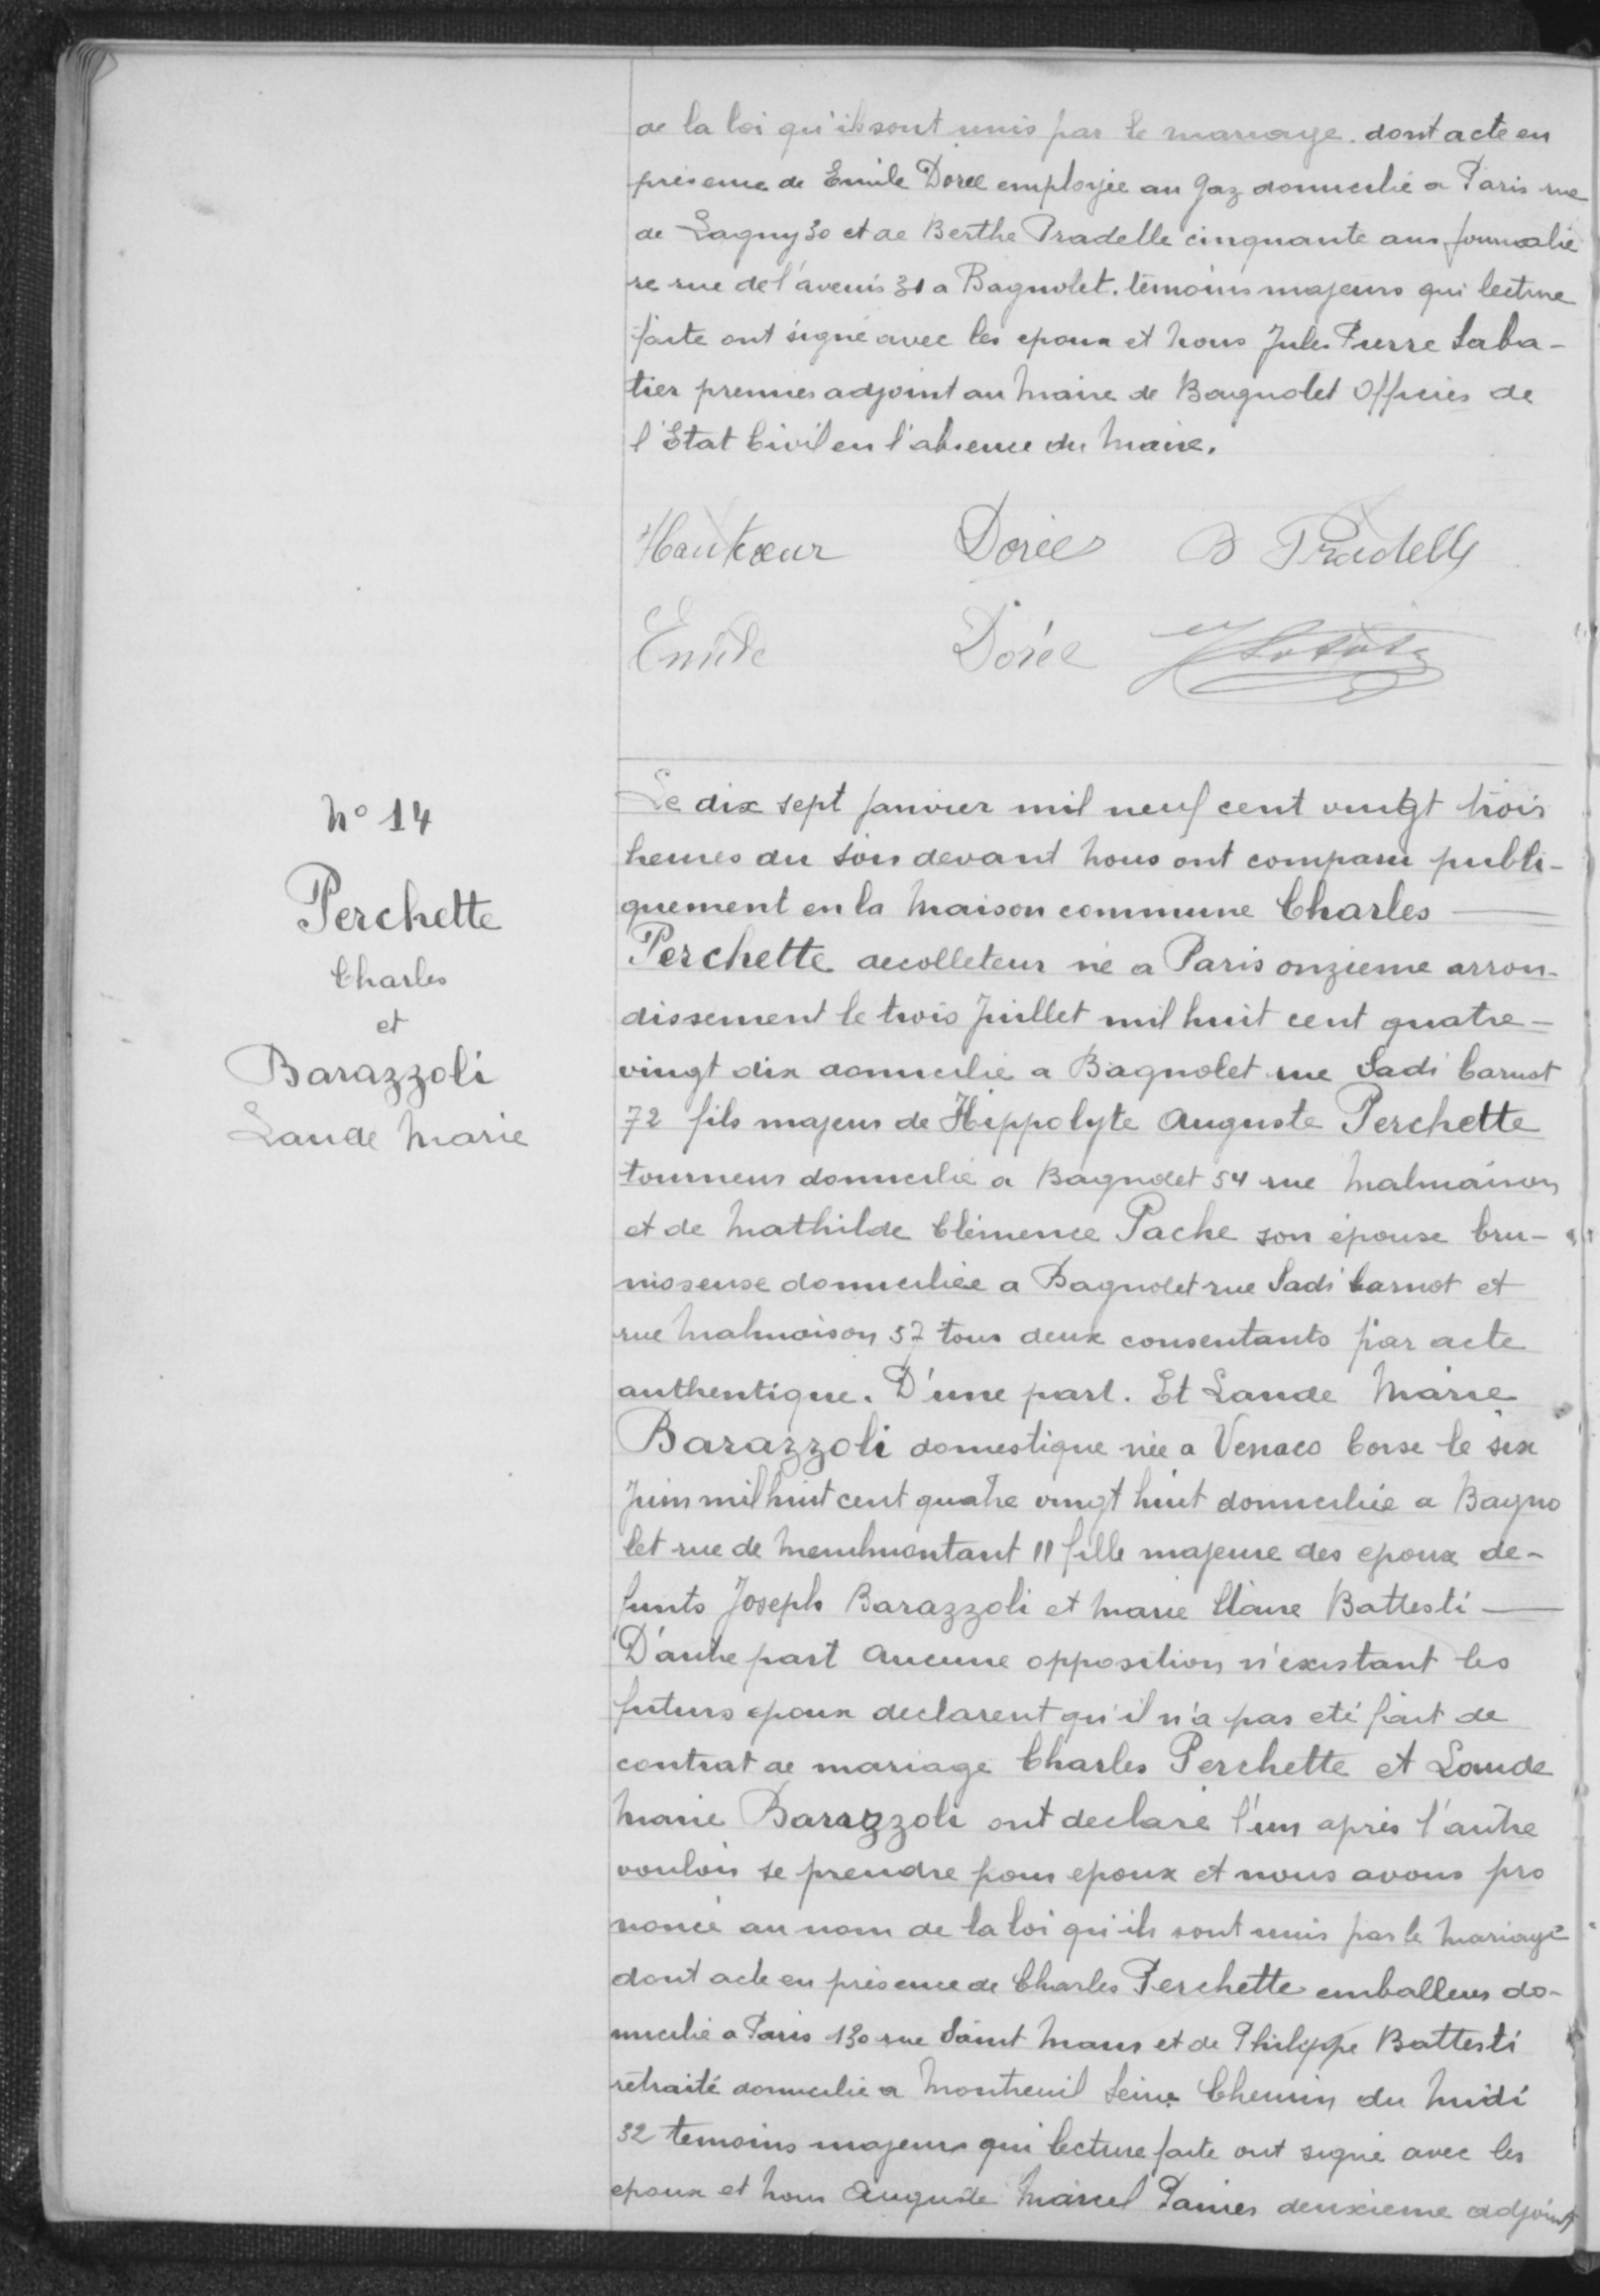a la loi qu'ils sont unis par le mariage, dont acte e
présence de Emile Doree emplloyé au gaz domicilié à Paris rue
de Laguy 30 et ae Berthe Pradelle cinquante ans journaliè
re rue de l'avenis 31 a Bagnolet, temoins majeurs qui lecture
faite ont signé avec les epoux et Nous Jules Pierre Saba-
tier premier adjoint au Maire de Bagnolet Officier de
l'Etat Civil en l'absence du Maire
N°14
Perchette
Charles
et
Barazzoli
Laude Marie
Le dix sept janvier mil neuf cent vingt trois
heures du sois devant nous ont comparu publi-
quement en la maison commune Charles
Perchette acollecteur né a Paris onzieme arron-
dissement le trois Juillet mil huit cent quatre-
vingt dix domicilié à Bagnolett rue Sadi Carnot
72 fils majeur de Hippolyte Auguste Perchette
tourneur domicilié a Bagnolet 54 rue malmaines
et de mathilde clémence Pache son épouse bru-
nisseuse domiciliée a Bagnolet rue Sadi Carnot et
rue malmaison 57 tous deux consentants par acte
authentique. D'une part. Et Laude Marie
Barazzoli domestique née a Venaco Corse le six
Juin mil huit cent quatre vingt huit domicilie a Bagno
let rue de Menilmontant 11 fille majeure des epoux de-
funts Joseph Barazzoli et Marie Claire Battesti
D'autre part aucune opposition n'existant les
futurs epoux declarent qu'il n'a pas été fait de
contrat de mariage Charles Perchette et Laude
Marie Barazzoli ont declaré l'un après l'autre
vouloir se prendre pour epoux et nous avons pro
noncé au nom de la loi qu'ils sont unis par le mariage
dont acte en présence de Charles Perchette emballeur do-
micilié à Paris 130 rue Saint Maur et de Philippe Battesti
retraité domicilié a Montreuil Seine Chemin du midi
32 temoins majeur qui lecture faite ont signé avec les
epoux et nous auguste Marcel Panies deuxieme adjoint
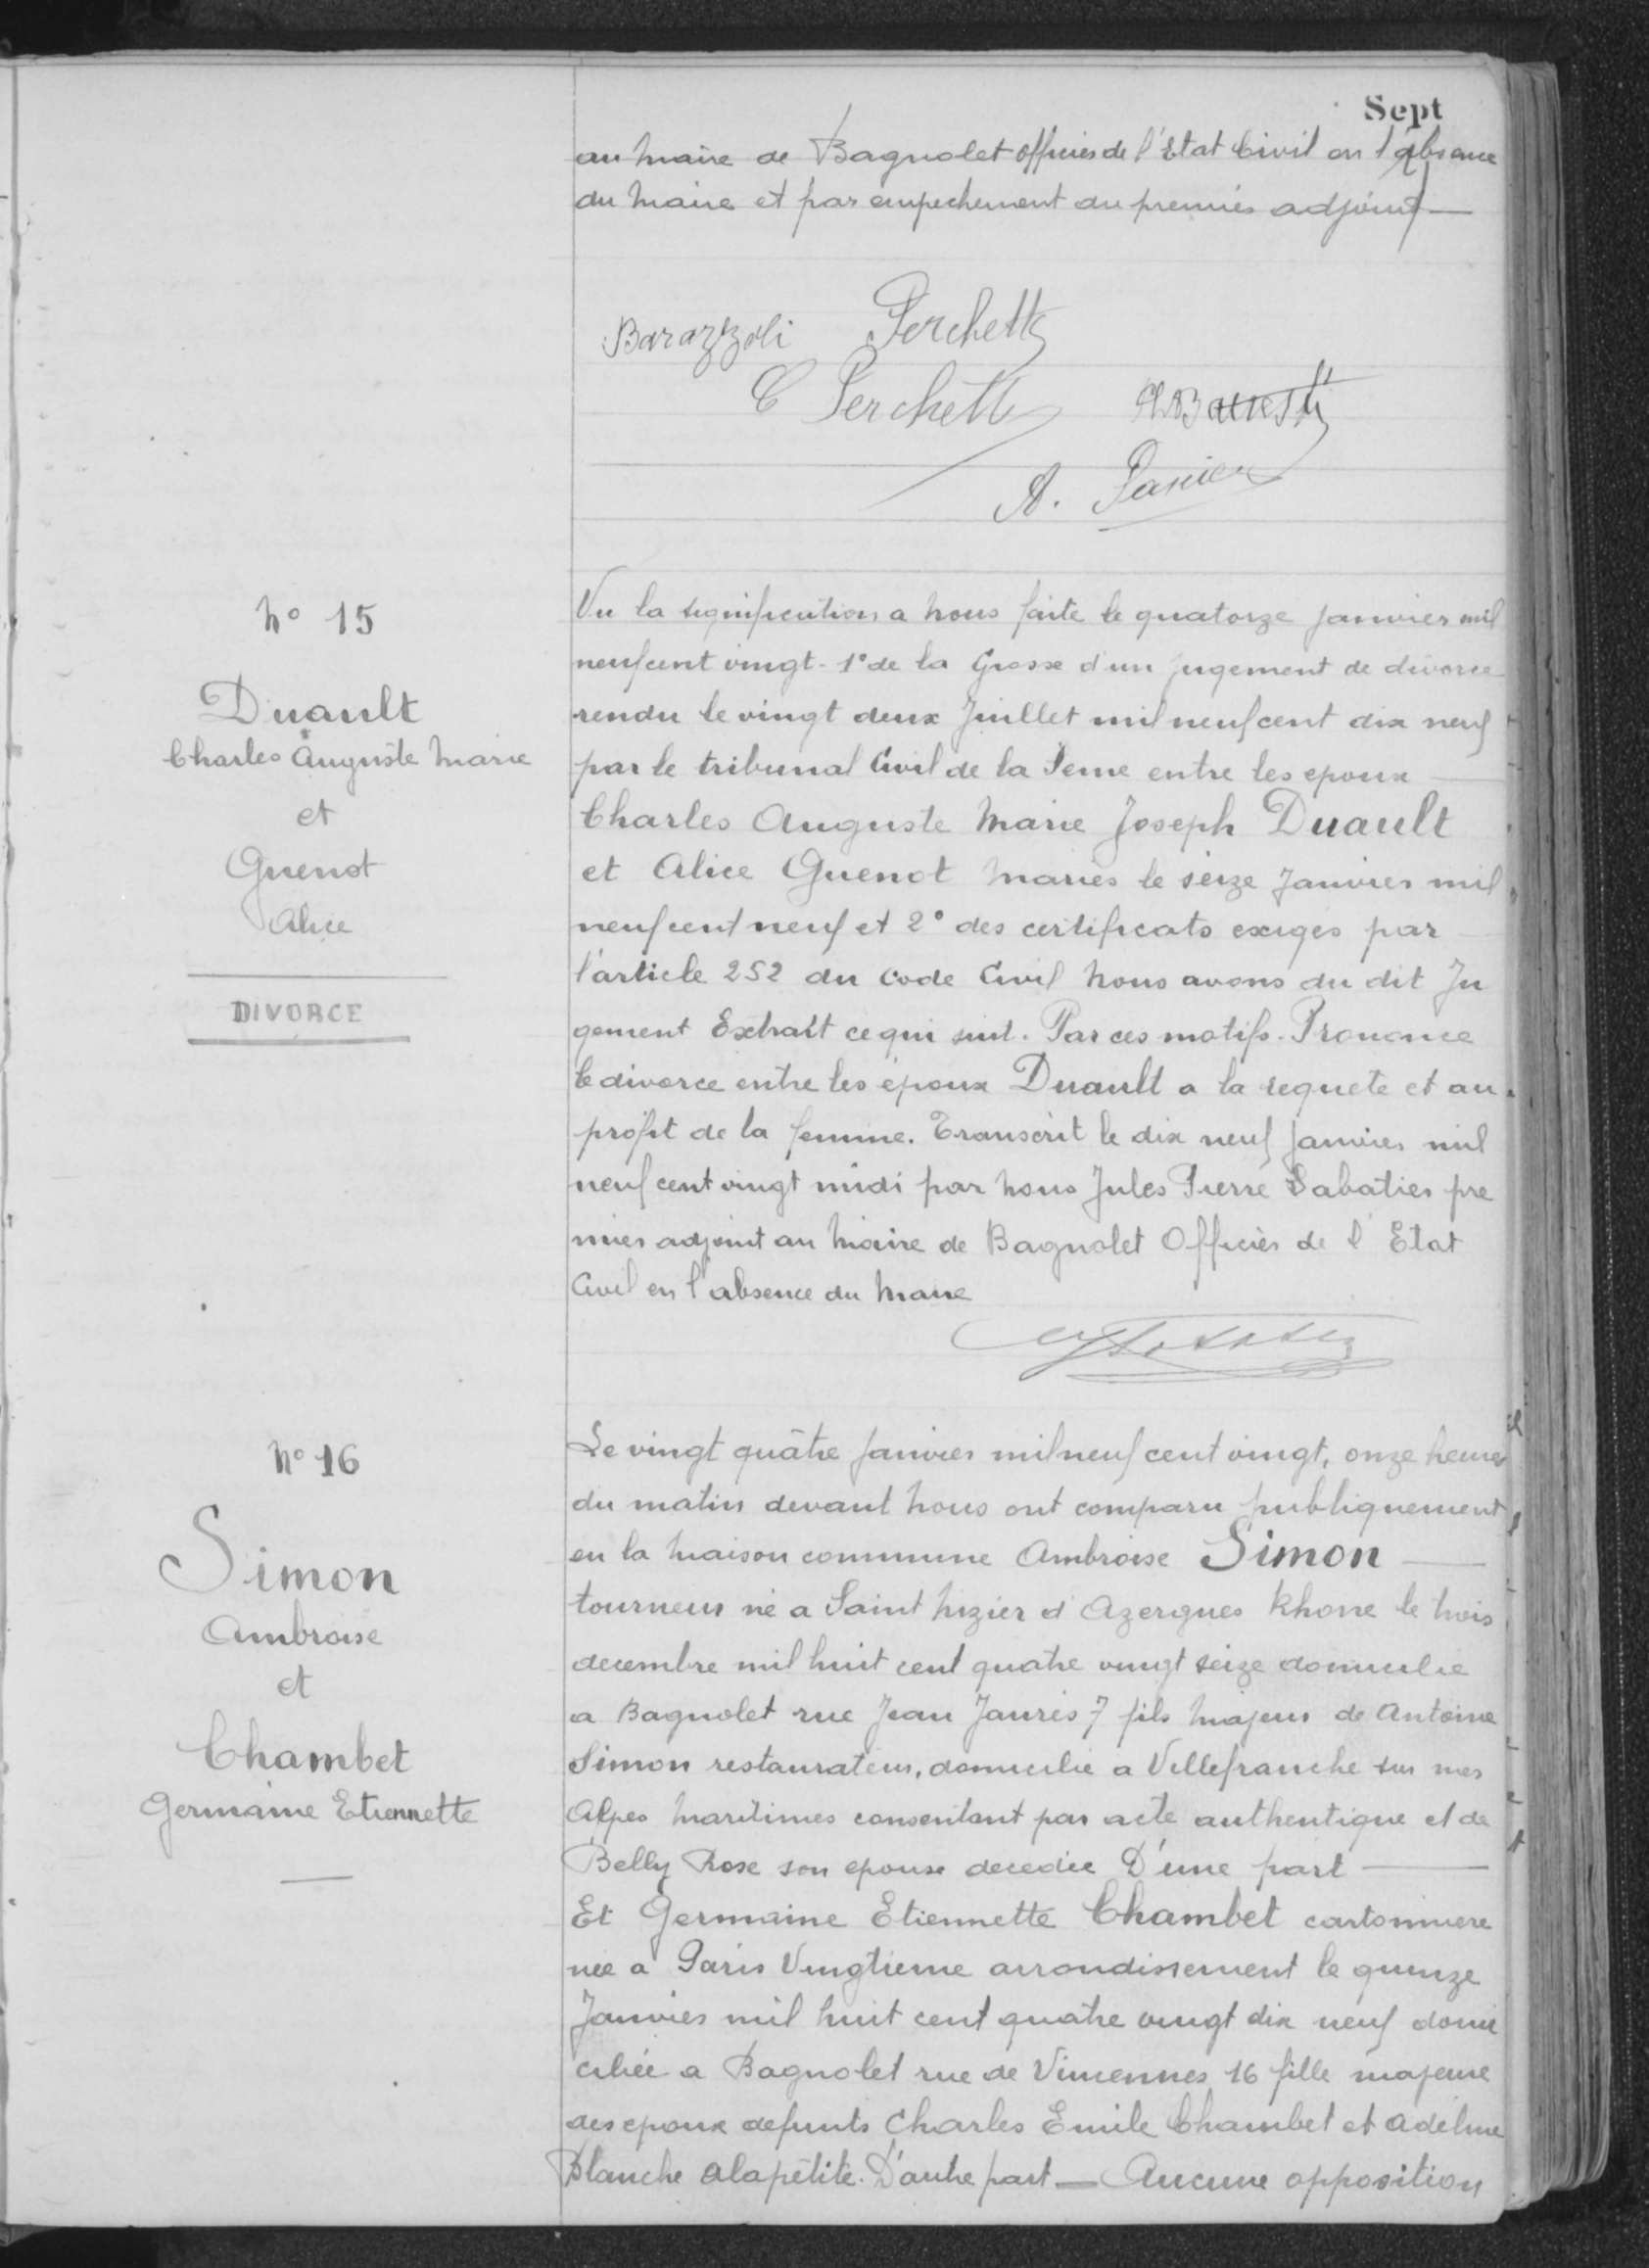au maire de Bagnolet officier de l'Etat Civil en l'absence
du maire et par empechement au premier adjoint
N°15
Duault
Charles Auguste Marie
et
Guenot
Alice
DIVORCE
Vu la signification a nous faite le quatorze janvier mil
neuf cent vingt-1° de la Grosse d'un jugement du divorce
rendu le vingt deux Juillet mil neuf cent dix neuf
par le tribunal civil de la Seine entre les epoux
Charles Auguste Maire Joseph Duault
et Alice Guenot mariés le seize Janvier mil
neuf cent neuf et 2° des certificats exigés par
l'article 252 du Code Civil nous avons du dit Ju
gement Extrait ce qui suit. Par ces motifs. Prononce
le divorce entre les époux Duault à la requête et au
profit de la femme. Transcrit le dix neuf Janvier mil
neuf cent vingt midi par nous Jules Pierre Sabatier pre
mier adjoint au Maire de Bagnolet Officier de l'Etat
Civil en l'absence du Maire
N°16
Simon
Ambroise
et
Chambet
Germaine Etienette
Le vingt quatre Janvier mil neuf cent vingt, onze heures
du matin devant Nous ont comparu publiquement
en la maison commune Ambroise Simon
tourneur né à Saint Nizier et Azergues Rhone le trois
decembre mil huit cent quatre vingt seize domicilié
a Bagnolet rue Jean Jaurès 7 fils majeur de Antoine
Simon restauration, domicilié à Villefranche sur mes
alpes maritimes consentant par acte authentique et de
Belly Rose son epouse decedée D'une part
Et Germaine Etiennette Chambet cartonniere
née a Paris vingtieme arrondissement le quinze
Janvier mil huit cent quatre vingt dix neuf domi
ciliée a Bagnolet rue de Vincennes 16 fille majeure
des epoux defunts Charles Emile Chambet et Adeline
Blanche Alapetite. D'autre part-Aucune opposition